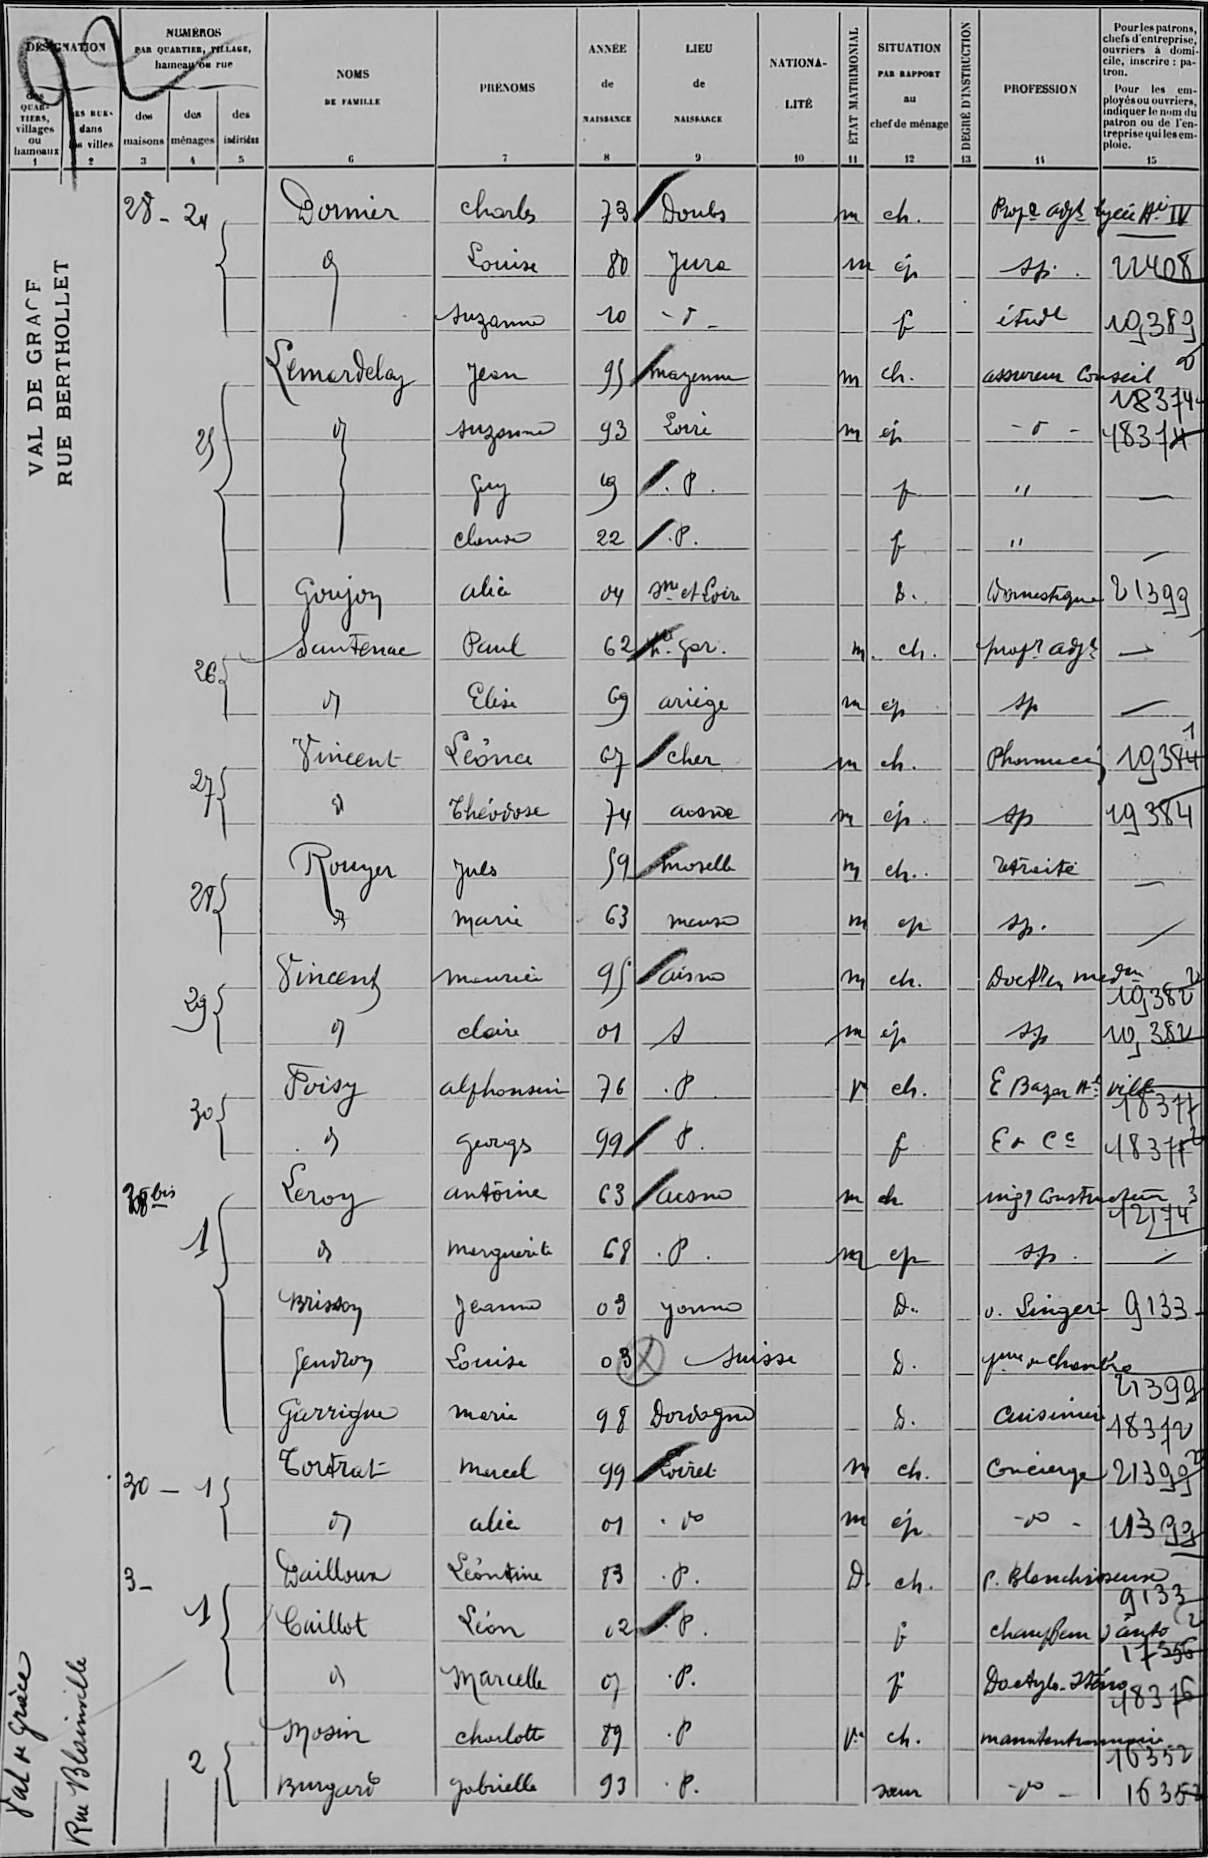Dormier/Charles/73/Doubs/¤/m/ch /¤/Prove adjt/lycée Hri IV
d/Louise/80/Jura/¤/m/ép/¤/sp/22408
d/Suzanne/10/d/¤/¤/f/¤/étude/19389
Lemardelay/Jean/95/Mayenne/¤/m/ch /¤/assureur conseil/!18374
d/Suzanne/93/Loire/¤/m/ép/¤/d/18374
d/Guy/10/P /¤/¤/f/¤/"/¤
d/Claude/22/P /¤/¤/f/¤/"/¤
Houjon/Alice/04/Sne et loire/¤/¤/d /¤/Domestique/21399
Sautenac/Paul/62/Hte Gar /¤/m/ch /¤/provr adj/¤
d/Elise/69/Ariège/¤/m/ép/¤/sp/¤
Vincnt/Léone/67/cher/¤/m/ch /¤/Pharmacien/19384
d/Théodore/74/Aisne/¤/m/ép/¤/sp/19384
Rouyer/Jules/59/Moselle/¤/m/ch /¤/retraité/¤
d/Marie/63/Meuse/¤/m/ep/¤/sp/¤
Vincent/Maurice/95/aisne/¤/m/ch /¤/Doct en medine/19382
d/Claire/01/S/¤/m/ép/¤/sp/19382
Foisy/alphonsine/76/P /¤/V/ch /¤/E Bazar Hl ville/!18377
d/Georges/99/P /¤/¤/f/¤/E de Cce/18377
Leroy/Antoine/63/aisne/¤/m/ch/¤/migr constructeur/!12174
d/Marguerite/68/P /¤/m/ep/¤/sp/¤
Brisson/jeanne/03/Yonne/¤/¤/d /¤/o lingère/9133
Gendron/Louise/03/Suisse/¤/¤/D /¤/fme de chambre/!21399
Garrigue/Marie/98/Dordogne/¤/¤/D /¤/cuisinière/18372
Tortrat/Marcel/99/Loiret/¤/m/ch /¤/concierge/21399
d/Alice/01/d°/¤/m/ép/¤/d°/11399
Dailloux/Léontine/83/P /¤/D/ch /¤/P Blanchisseuse/!9133
Caillot/Léon/02/P /¤/¤/f/¤/chauffeur d'auto/!17356
d°/MArcelle/07/P /¤/¤/f/¤/Dactylo Sténo/!18376
Mosin/Charloette/89/P/¤/Ve/ch /¤/manutentionnaire/!16352
Burgard/Gabrielle/93/P /¤/¤/soeur/¤/d°/16352
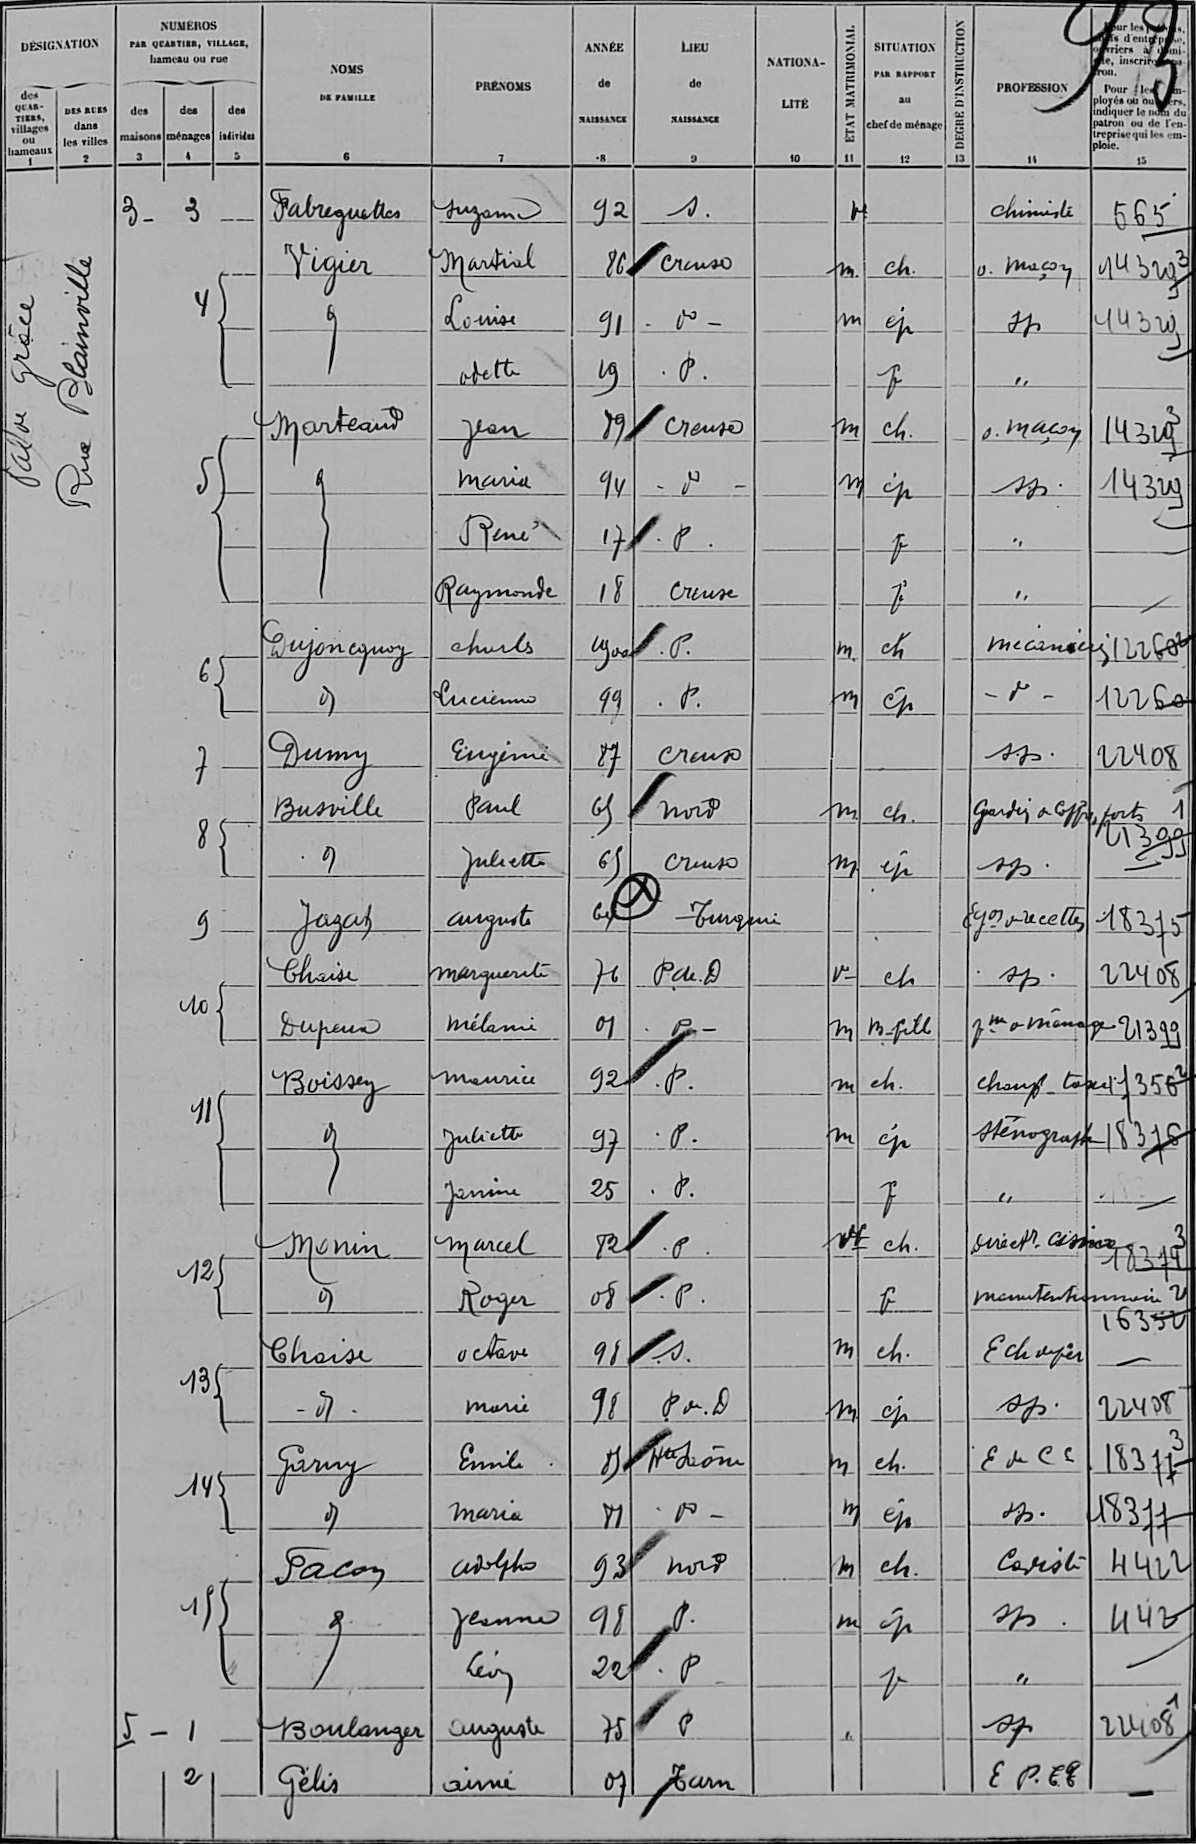Fabreguettes/Suzanne/92/S/¤/Ve/¤/¤/chimiste/565
Vigier/Martial/96/Creuse/¤/m /ch /¤/o maçon/14329
d°/Louise/91/d°/¤/m/ép/¤/sp/14329
d°/odette/19/P /¤/¤/f/¤/"/¤
Marteau/Jean/89/Creuse/¤/M/ch /¤/o maçon/14329
d°/Maria/94/d°/¤/M/ép/¤/sp/14329
d°/René/17/-P-/¤/¤/f/¤/"/¤
d°/Raymonde/18/Creuse/¤/¤/f/¤/"/¤
Dujoncquoy/charles/1900/P /¤/m/ch/¤/mécanicien/12260
d°/Lucienne/99/P /¤/m/ép/¤/d/12260
Dumy/Eugénie/87/Creuse/¤/¤/¤/¤/sp/22408
Busville/Paul/65/Nord/¤/m/ch /¤/Gardien de coffres forts/!21399
d°/Juliette/65/Creuse/¤/m/ép/¤/sp/¤
Jazah/auguste/64/Turquie/¤/¤/¤/¤/E gon de recettess/18375
Chaise/marguerite/76/P de D/¤/Ve/ch/¤/sp /22408
Dupeux/Mélanie/01/P-/¤/m/B-fille/¤/fme de ménage/21399
Boissey/Maurice/92/P /¤/m/ch /¤/chauf taxi/17356
d°/Juliette/97/P /¤/m/ép/¤/sténographe/18376
d°/Janine/25/P /¤/¤/f/¤/"/¤
Monin/Marcel/82/P /¤/Vf/ch /¤/Directr cisime/!18379
d°/Roger/08/P /¤/¤/f/¤/manutentionnaire/!16352
Chaise/octave/98/S /¤/m/ch /¤/E chauf/¤
-d°d/marie/98/P de D/¤/m/ép/¤/sp /22408
Garny/Emile/85/Hte Saône/¤/m/ch /¤/E de Ce/18377
d°/Maria/81/d°/¤/M/ép/¤/sp /18377
Facon/Adolphe/93/Nord/¤/M/ch /¤/Caviste/4422
d/Jeanne/98/P /¤/m/ép/¤/sp/442
d/Léon/22/P /¤/¤/f/¤/"/¤
Boulanger/Auguste/75/P/¤/¤/¤/¤/sp/22408
Gélis/aimée/07/Tarn/¤/¤/¤/¤/E PTT/¤